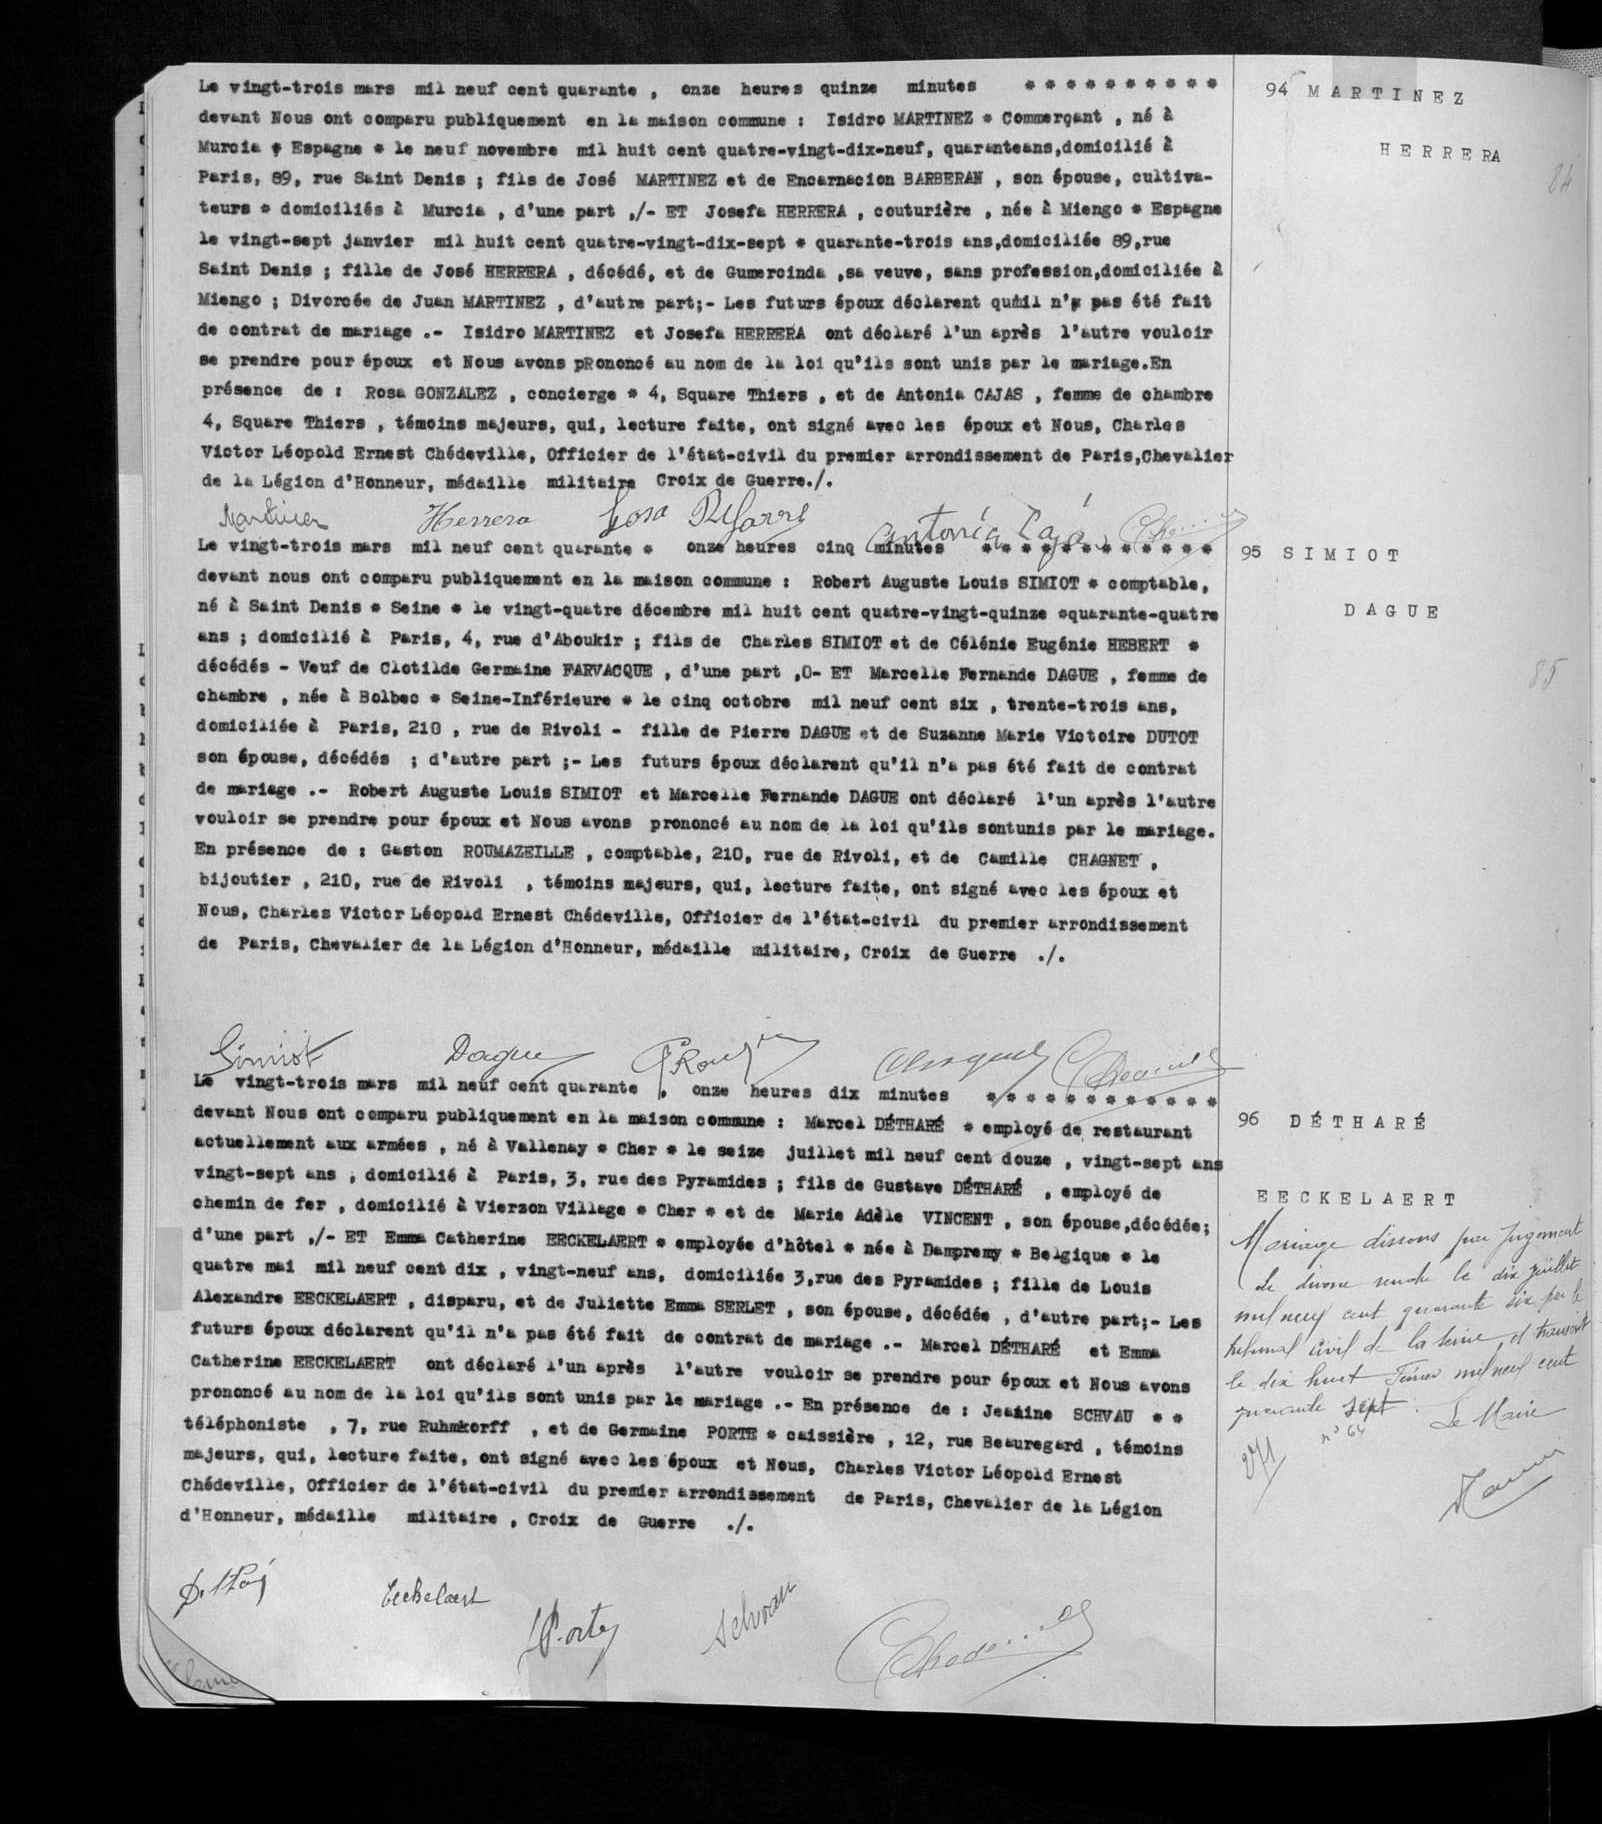Le vingt-trois mars mil neuf cent quarante, onze heures quinze minutes ****
devant Nous ont comparu publiquement en la maison commune: Isidro MARTINEZ * Commerçant, né à
Muroia * Espagne * le neuf novembre mil huit cent quatre-vingt-dix-neuf, quarante ans, domicilié à
Paris, 89, rue Saint Denis; fils de José MARTINEZ et de Encarnacion BARBERAN, son épouse, cultiva-
teurs * domiciliés Muroia, d'une part ,/-ET Josefa HERRERA, couturière, née à Miengo * Espagne
le vingt-sept janvier mil huit cent quatre-vingt-dix-sept * quarante-trois ans, domiciliée 89, rue
Saint Denis; fille de José HERRERA, décédé, et de Gumercinda, sa veuve, sans profession, domiciliée à
Miengo; Divorcée de Juan MARTINEZ, d'autre part ;-Les futurs époux déclarent qu'il n'a pas été fait
de contrat de mariage .-Isidro MARTINEZ et Joseph HERRERA ont déclaré l'un après l'autre vouloir
se prendre pour époux et Nous avons prononcé au nom de la loi qu'ils sont unis par le mariage. En
présence de: Rosa GONZALEZ, concierge * 4, Square Thiers, et de Antonia CAJAS, femme de chambre
4, Square Thiers, témoins majeurs, qui, lecture faite, ont signé avec les époux et Nous, Charles
Victor Léopold Ernest Chédeville, Officier de l'état-civil du premier arrondissement de Paris, Chevalier
de la Légion d'Honneur, médailler militaire Croix de Guerre ./.
Le vingt-trois mars mil neuf cent quarante * onze heures cinq minutes ****
devant nous ont comparu publiquement en la maison commune: Robert Auguste Louis SIMIOT * comptable,
né à Saint Denis * Seine * le vingt-quatre décembre mil huit cent quatre-vingt-quinze * quarante-quatre
ans; domicilié à Paris, 4, rue d'Aboukir; fils de Charles SIMIOT et de Célénie Eugénie HEBERT *
décédés-Veuf de Clotilde Germaine FARBACQUE, d'une part, 0-ET Marcelle Fernande DAGUE, femme de
chambre, née à Bolbec * Seine-Inférieure * le cinq octobre mil neuf cent six, trente-trois ans,
domiciliée à Paris, 210, rue de Rivoli-fille de Pierre DAGUER et de Suzanne Marie Victoire DUTOT
son épouse, décédés; d'autre part ;-Les futurs époux déclarent qu'il n'a pas été fait de contrat
de mariage .-Robert Auguste Louis SIMIOT et Marcelle Fernande DAGUE ont déclaré l'un après l'autre
vouloir se prendre pour époux et Nous avons prononcé au nom de la loi qu'ils sont unis par le mariage.
En présence de: Gaston ROUMAZEILLE, comptable, 210, rue de Rivoli, et de Camille CHAGNET
bijoutier, 210, rue de Rivoli, témoins majeurs, qui, lecture faite, ont signé avec les époux et
Nous, Charles Victor Léopold Ernest Chédeville, Officier de l'état-civil du premier arrondissement
de Paris, Chevalier de la Légion d'Honneur, médaille militaire, Croix de Guerre ./.
Le vingt-trois mars mil neuf cent quarante, onze heures dix minutes ****
devant Nous ont comparu publiquement en la maison commune: Marcel DETHARE * employé de restaurant
actuellement aux armées, né à Vallenay * Cher * le seize juillet mil neuf cent douze, vingt-sept ans
vingt-sept ans, domicilié à Paris, 3, rue des Pyramides; fils de Gustave DETHARE, employé de
chemin de fer, domicilié à Vierzon Village * Cher * et de Marie Adèle VINCENT, son épouse, décédée;
d'une part ,/-ET Emma Catherine EECKELAERT * employée d'hôtel * née à Dampremy * Belgique * le
quatre mai mil neuf cent dix, vingt-neuf ans, domiciliée 3, rue des Pyramides; fille de Louis
Alexandre EECKELAERT, disparu, et de Juliette Emma SERLET, son épouse, décédée, d'autre part ;-Les
futurs époux déclarent qu'il n'a pas été fait de contrat de mariage .-Marcel DETHARE et Emma
Catherine EECKELAERT ont déclaré l'un après l'autre vouloir se prendre pour époux et Nous avons
prononcé au nom de la loi qu'ils sont unis par le mariage .-En présence de: Jeanine SCHVAU **
téléphoniste, 7, rue Ruhmkorff, et de Germaine PORTE * caissière, 12, rue Beauregard, témoins
majeurs, qui, lecture faite, ont signé avec les époux et Nous, Charles Victor Léopold Ernest
Chédeville, Officier de l'état-civil du premier arrondissement de Paris, Chevalier de la Légion
d'Honneur, médaille militaire, Croix de Guerre ./.
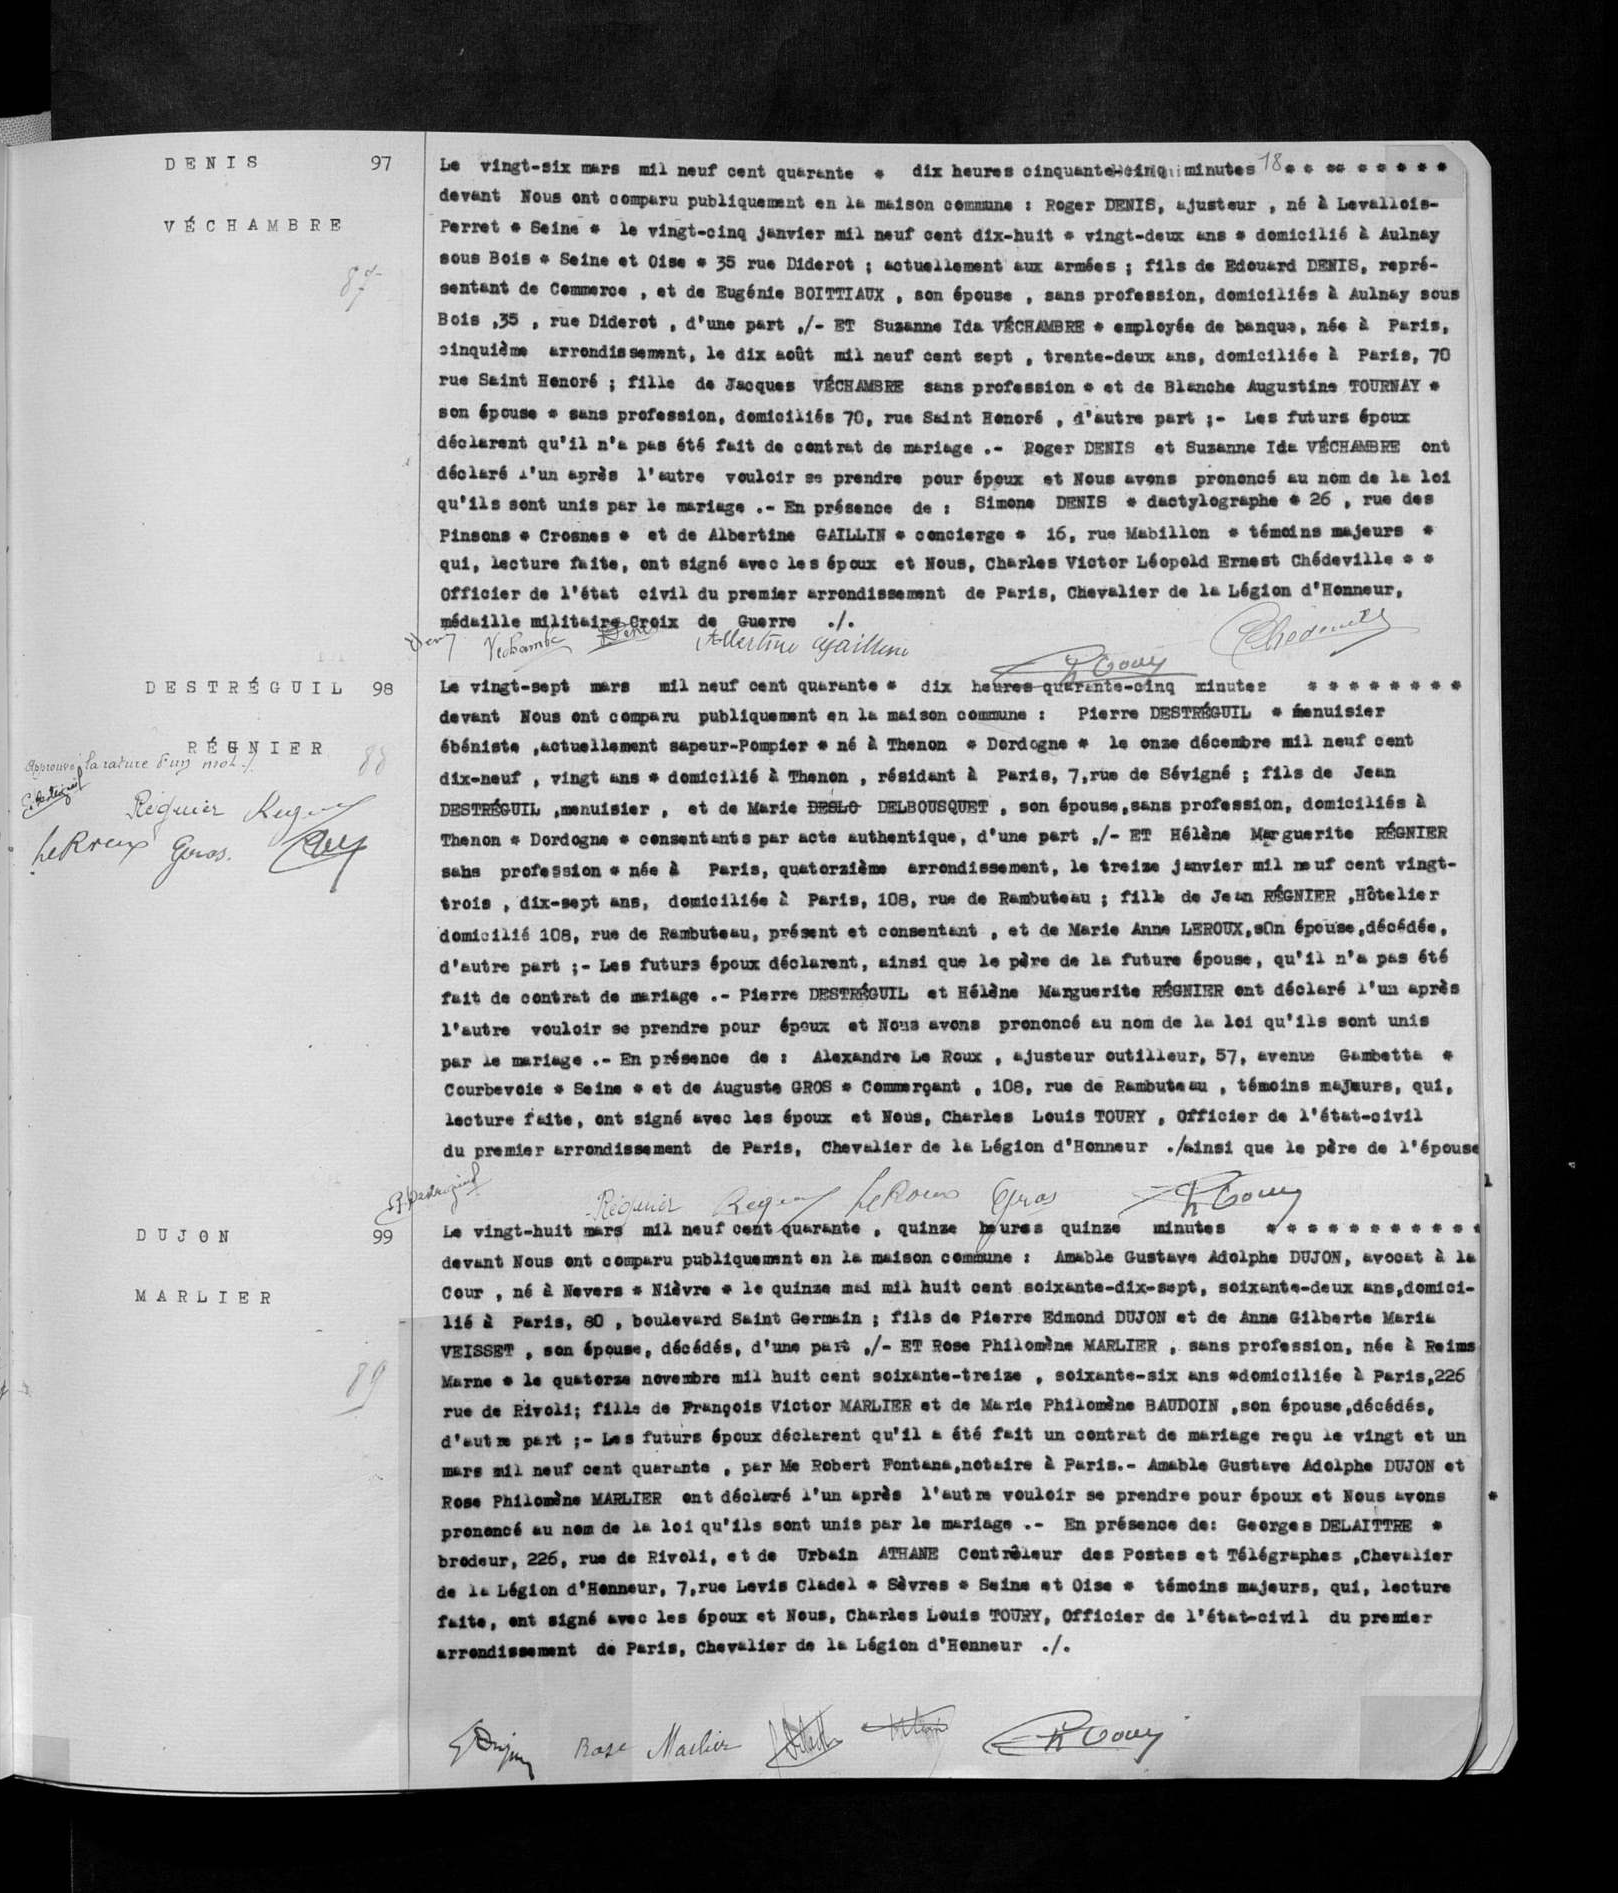Le vingt-six mars mil neuf cent quarante * dix heures cinquante-cinq minutes ****
devant Nous ont comparu publiquement en la maison commune: Roger DENIS, ajusteur, né à Levallois-
Perret * Seine * le vingt-cinq janvier mil neuf cent dix-huit * vingt-deux ans * domicilié à Aulnay
sous Bois * Seine et Oise * 35 rue Diderot; actuellement aux armées; fils de Edouard DENIS, repré-
sentant de Commerce, et de Eugénie BOITTIAUX, son épouse, sans profession, domiciliés à Aulnay sous
Bois, 35, rue Diderot, d'une part ,/-ET Suzanne Ida VECHAMBRE * employée de banque, née à Paris,
cinquième arrondissement, le dix août mil neuf cent sept, trente-deux ans, domiciliée à Paris, 70
rue Saint Honoré; fille de Jacques VECHAMBRE sans profession * et de Blanche Augustine TOURNAY *
son épouse * sans profession, domiciliés 70, rue Saint Honoré, d'autre part ;-Les futurs époux
déclarent qu'il n'a pas été fait de contrat de mariage .-Roger DENIS et Suzanne Ida VECHAMBRE ont
déclaré l'un après l'autre vouloir se prendre pour époux et Nous avons prononcé au nom de la loi
qu'ils sont unis par le mariage .-En présence de: Simone DENIS * dactylographe * 26, rue des
Pinsons * Crosnes * et de Albertine GAILLIN * concierge * 16, rue Mabillon * témoins majeurs *
qui, lecture faite, ont signé avec les époux et Nous, Charles Victor Léopold Ernest Chédeville **
Officier de l'état civil du premier arrondissement de Paris, Chevalier de la Légion d'Honneur,
médaille militaire Croix de Guerre ./.
Le vingt-sept mars mil neuf cent quarante * dix heures quarante-cinq minutes ****
devant Nous ont comparu publiquement en la maison commune: Pierre DESTREGUIL * ménuisier
ébéniste, actuellement sapeur-Pompier * né à Thenon * Dordogne * le onze décembre mil neuf cent
dix-neuf, vingt ans * domicilié à Thenon, résidant à Paris, 7, rue de Sévigné; fils de Jean
DESTREGUIL, menuisier, et de Marie DESLO DELBOUSQUET, son épouse, sans profession, domiciliés à
Thenon * Dordogne * consentants par acte authentique, d'une part ,/-ET Hélène Marguerite REGNIER
sans profession * née à Paris, quatorzième arrondissement, le treize janvier mil neuf cent vingt-
trois, dix-sept ans, domiciliée à Paris, 108, rue de Rambuteau; fille de Jean REGNIER, Hôtelier
domicilié 108, rue de Rambuteau, présent et consentant, et de Marie Anne LEROUX, son épouse, décédée,
d'autre part ;-Les futurs époux déclarent, ainsi que le père de la future épouse, qu'il n'a pas été
fait de contrat de mariage .-Pierre DESTREGUIL et Hélène Marguerite REGNIER ont déclaré l'un après
l'autre vouloir se prendre pour époux et Nous avons prononcé au nom de la loi qu'ils ont unis
par le mariage .-En présence de: Alexandre Le Roux, ajusteur outilleur, 57, avenue Gambetta *
Courbevoie * Seine * et de Auguste GROS * Commerçant, 108, rue de Rambuteau, témoins majeurs, qui,
lecture faite, ont signé avec les époux et Nous, Charles Louis TOURY, Officier de l'état-civil
du premier arrondissement de Paris, Chevalier de la Légion d'Honneur ./ ainsi que le père de l'épouse
Le vingt-huit mars mil neuf cent quarante, quinze heures quinze minutes ****
devant Nous ont comparu publiquement en la maison commune: Amable Gustave Adolphe DUJON, avocat à la
Cour, né à Nevers * Nièvre * le quinze mai mil huit cent soixante-dix-sept, soixante-deux ans, domici-
lié à Paris, 80, boulevard Saint Germain; fils de Pierre Edmond DUJON et de Anne Gilberte Maria
VEISSET, son épouse, décédés, d'une part ,/-ET Rose Philomène MARLIER, sans profession, née à Reims
Marne * le quatorze novembre mil huit cent soixante-treize, soixante-six ans * domiciliée à Paris, 226
rue de Rivoli; fille de François Victor MARLIER et de Marie Philomène BAUDOUIN, son épouse, décédés,
d'autre part ;-Les futurs époux déclarent qu'il a été fait un contrat de mariage reçu le vingt et un
mars mil neuf cent quarante, par Me Robert Fontana, notaire à Paris .-Amable Gustave Adolphe DUJON et
Rose Philomène MARLIER ont déclaré l'un après l'autre vouloir se prendre pour époux et Nous avons
prononcé au nom de la loi qu'ils ont unis par le mariage .-En présence de: Georges DELAITTRE *
brodeur, 226, rue de Rivoli, e t de Urbain ATHANE Contrôleur des Postes et Télégraphes, Chevalier
de la Légion d'Honneur, 7, rue Levis Cladel * Sèvre * Seine et Oise * témoins majeurs, qui, lecture
faite, ont signé avec les époux et Nous, Charles Louis TOURY, Officier de l'état-civil du premier
arrondissement de Paris, Chevalier de la Légion d'Honneur ./.